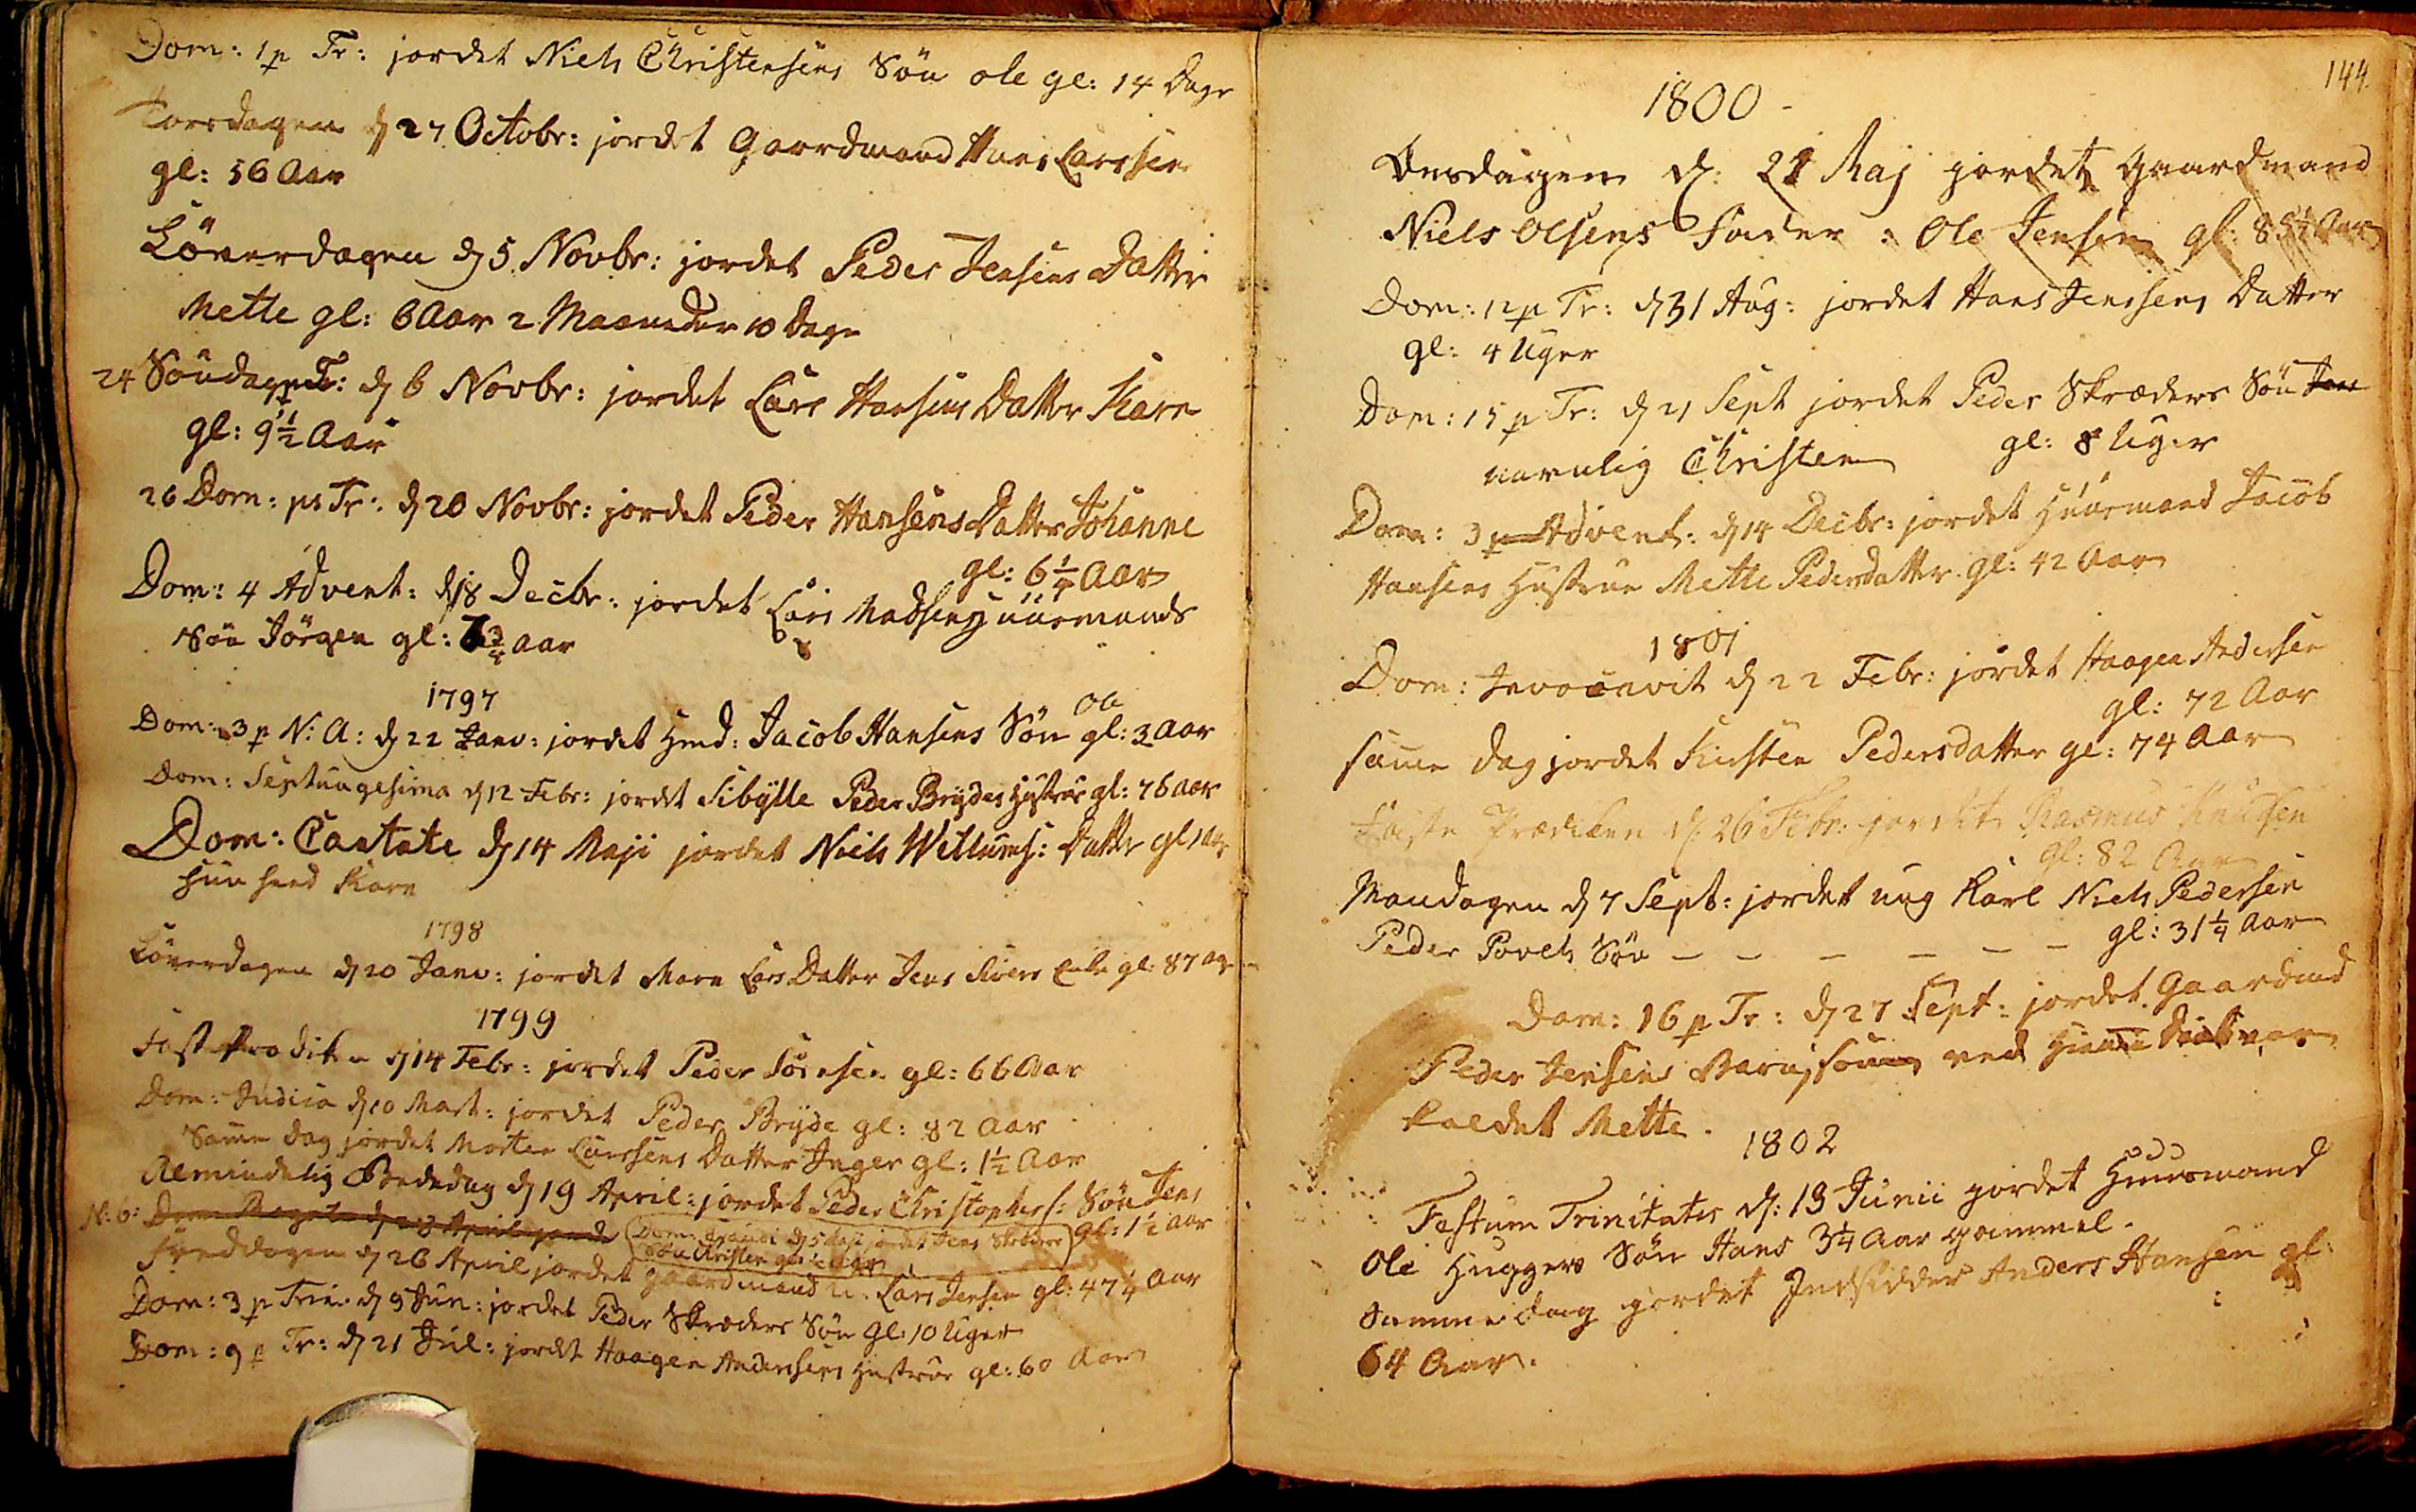Dom: 1 p Tr: jordet Niels Christensens Søn Ole gl: 14 Dage
Torsdagen d 27 Octobr: jordet Gaardmand Hans Larssen
gl: 56 Aar
Løverdagen d 5 Novbr: jordet Peder Jensens Datter
Mette gl: 6 Aar 2 Maaneder 10 Dage
24 Søndag p Tr: d 6 Novbr: jordet Lars Hansens Datter Karn
gl: 9½ Aar
26 Dom: p: Tr: d 20 Novbr: jordet Peder Hansens Datter Johanne
gl: 6 ¼ Aar
Dom: 4 Advent: d 18 Decbr: jordet Lars Madsen Huusmands
Søn Jørgen gl: 7 ¾ Aar
1797
Ole
Dom: 3 p N:A: d 22 Janu: jordet Hmd: Jacob Hansens Søn gl: 3 Aar
Dom: Septuagesima d 12 Febr: jordet Sibylle Peder Brydes Hustrue gl:76 Aar
Dom: Cantate d 14 Maji jordet Niels Willums: Datter gl 1 Aar
Hun heed Karn
1798
Løverdagen d 20 Janu: jordet Marn Lars Datter Jens Sivers Enke gl: 87 Aar
1799
Faste Prædiken d 14 Febr: jordet Peder Sørensen gl: 66 Aar
Dom: Judica d 10 Mart: jordet Peder Bryde gl: 82 Aar
Samme Dag jordet Morten Larssens Datter Inger gl: 1½ Aar
Almindelig Bededag d 19 April: jordet Peder Christophers: Søn Jens
gl: 1½ Aar
N:b: Dom: Rogate d 28 April jorde
Dom Exaudi d 5 Maji jordet Jens Skræders
Søn Christen gl: ½ Aar
Freddagen d 26 April jordet Gaardmand U: Lars Jensen gl: 47 ¼ Aar
Dom: 3 p Trin: d 9 Jun: jordet Peder Skræders Søn gl: 10 Uger
Dom: 9 p Tr: d 21 Jul: jordet Haagen Andersens Hustrue gl: 60 Aar
144.
1800
Onsdagen d: 21 Maj jordet Gaardmand
Niels Olsens Fader: Ole Jensen gl: 85½ Aar
Dom: 12 p Tr: d 31 Aug: jordet Hans Jenssens Datter
gl: 4 Uger
Dom: 15 p Tr: d 21 Sept jordet Peder Skræders Søn Jens
navnlig Christen gl: 8 Uger
Dom: 3 p Advent: d 14 Decebr: jordet Huusmand Jacob
Hansens Hustrue Mette Pedersdatter. gl: 42 Aar
1801
Dom: Invocavit d 22 Febr: jordet Haagen Andersen
gl: 72 Aar
samme dag jordet Kirsten Pedersdatter gl: 74 Aar
Faste Prædiken d: 26 Febr: jordet Rasmus Knudsen
gl: 82 Aar
Mandagen d 7 Sept: jordet ung Karl Niels Pedersen
Peder Povels Søn 
gl: 31 ¼ Aar
Dom: 16 p Tr: d 27 Sept: jordet Gaardmd
Peder Jensens Barn, som ved Hiemme Daab var
kaldet Mette.
1802
Festum Trinitatis d: 13 Junii jordet Huusmand
Ole Huggers Søn Hans 3 ¼ Aar gammel.
Samme Dag jordet Indsidder Anders Hansen gl:
64 Aar.
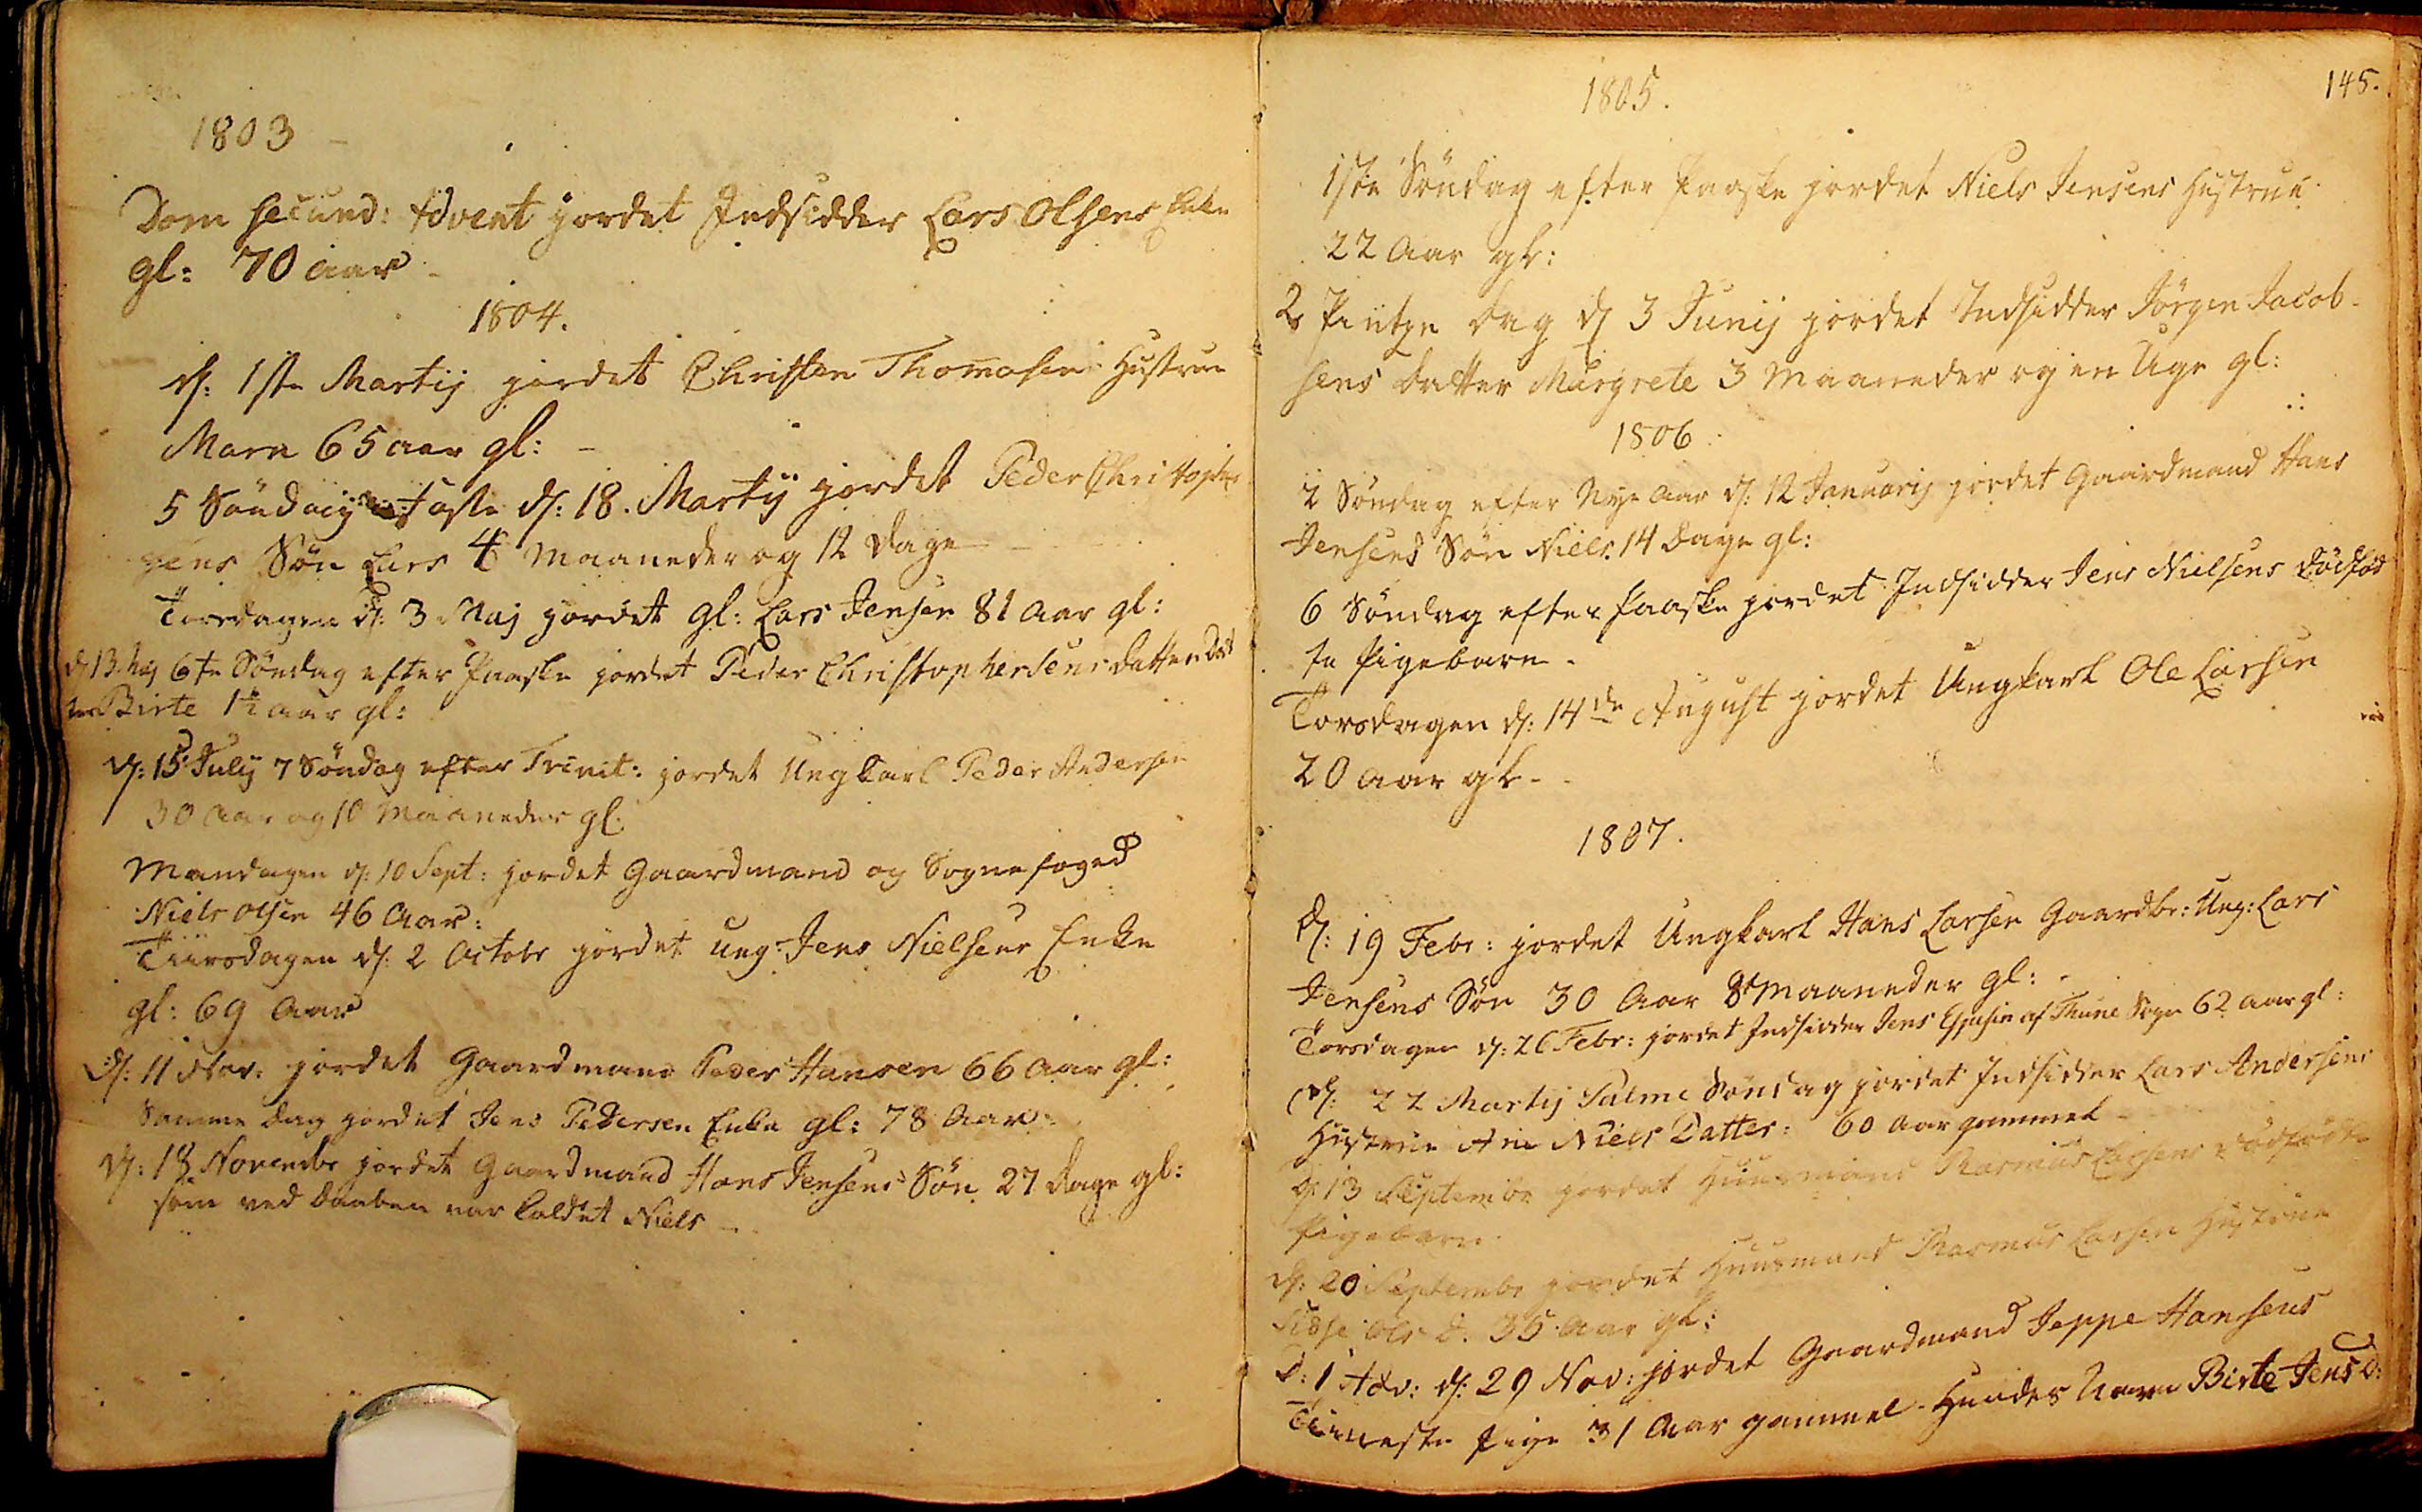1803
Dom secund: Advent jordet Indsidder Lars Olsens Enke
gl: 70 Aar.
1804.
d: 1ste Martij jordet Christen Thomasens Hustrue
Marn 65 Aar gl:
5 Søndag i Faste d: 18. Martij jordet Peder Christopher
sens Søn Lars 4 Maaneder og 12 Dage
Torsdagen d: 3 maj jordet gl: Lars Jensen 81 Aar gl:
d 13 Maj 6te Søndag efter Paaske jordet Peder Christophersens Datter Dat
ter Birte 1½ Aar gl:
d: 15 Julij 7 Søndag efter Trinit: jordet Ungkarl Peder Andersen
30 Aar og 10 Maaneder gl:
Mandagen d: 10 Sept: jordet Gaardmand og Sognefoged
Niels Olsen 46 Aar:
Tiirsdagen d: 2 Octobr jordet Ung Jens Nielsens Enke
gl: 69 Aar
d: 11 Nov: jordet Gaardmand Peder Hansen 66 Aar gl:
Samme Dag jordet Jens Pedersen Enke gl: 78 Aar
d: 18 Novembr jordet Gaardmand Hans Jensens Søn 27 Dage gl:
som ved Daaben var kaldet Niels
145.
1805
1ste Søndag efter Paaske jordet Niels Jensens Hustrue
22 Aar gl:
2 Pintze Dag d 3 Junij jordet Indsidder Jørgen Jacob¬
sens Datter Margrete 3 Maaneder og en Uge gl:
1806
2 Søndag efter Nye Aar d: 12 Januarij jordet Gaardmand Hans
Jensens Søn Niels 14 dage gl:
6 Søndag efter Paaske jordet Indsidder Jens Nielsens dødfød
te Pigebarn.
Torsdagen d: 14de August jordet Ungkarl Ole Larsen
20 Aar gl.
1807.
d: 19 Febr: jordet Ungkarl Hans Larsen Gaardbr: Ung: Lars
Jensens Søn 30 Aar 8 Maaneder gl:
Torsdagen d:26 Febr:jordet Indsidder Jens Espesin af Thune Sogn 62 Aar gl:
d: 22 Martij Palme Søndag jordet Indsidder Lars Andersens
Hustrue, Ane Niels Datter: 60 Aar gammel.
d: 13 Septembr jordet Huusmand Rasmus Larsens dødfødte
Pigebarn.
d. 20 Septembr jordet Huusmand Rasmus Larsen Hustrue
Sidse Ols D: 35 Aar gl:
D: 1 Adv: d: 29 Nov: jordet Gaardmand Jeppe Hansens
Tieneste Pige 31 Aar gammel. Hendes Navn Birte Jens D: